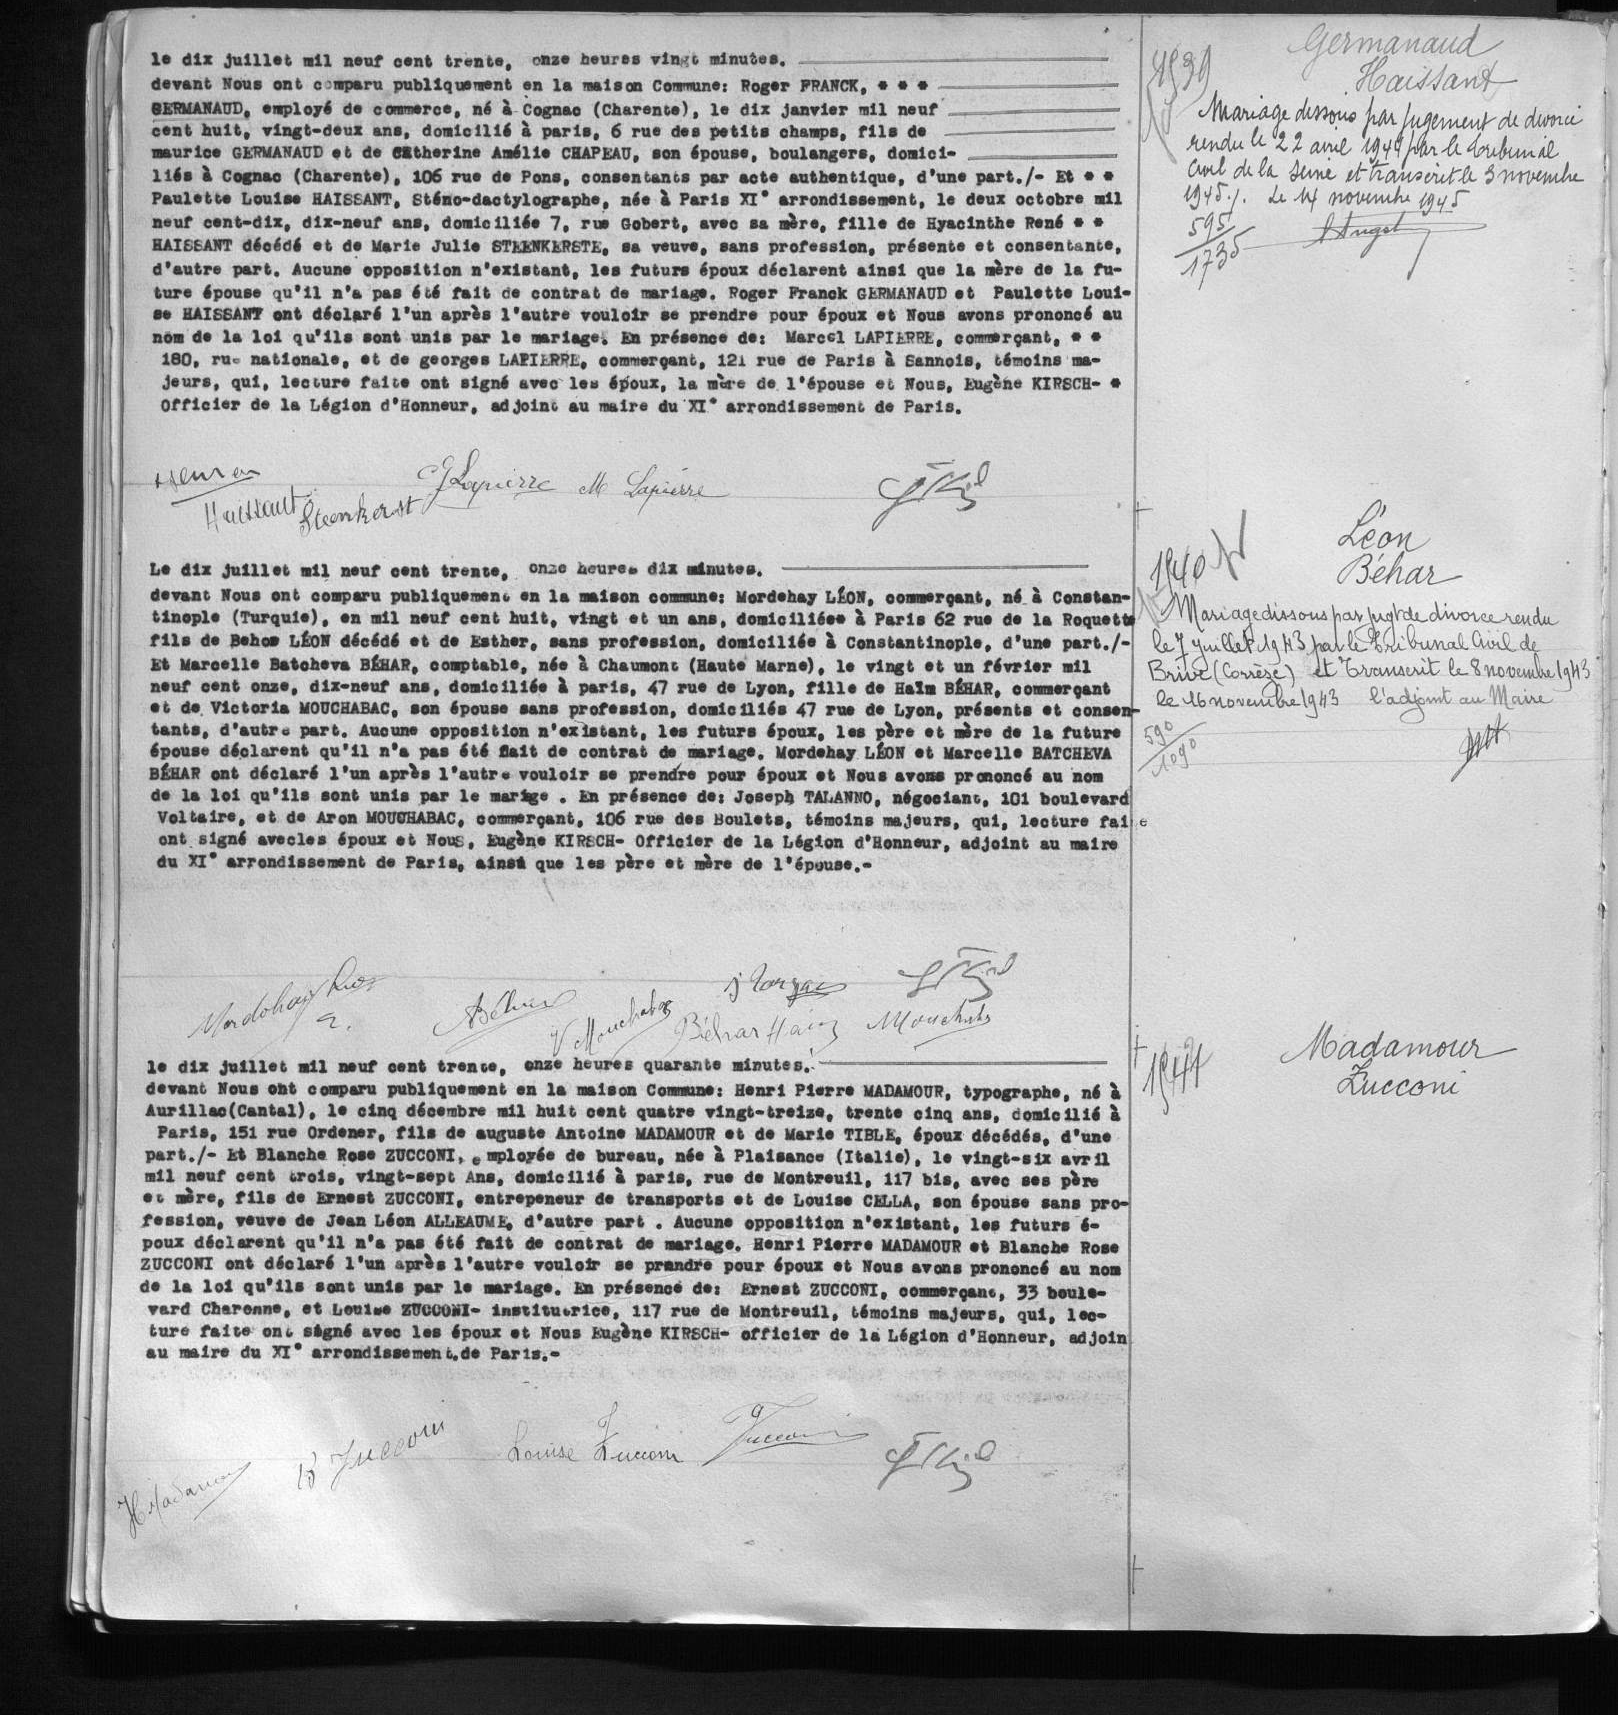le dix juillet mil neuf cent trente, onze heures vingt minutes .-
devant Nous ont comparu publiquement en la maison Commune: Roger FRANCK, ***-
GERMANAUD, employé de commerce, né à Cognac (Charente), le dix janvier mil neuf -
cent huit, vingt-deux ans, domicilié à paris, 6 rue des petits champs, fils de -
maurice GERMANAUD et de CEtherine Amélie CHAPEAU, son épouse, boulanger, domici -
liés à Cognac (Charente), 106 rue de Pons, consentants par acte authentique, d'une part ./-Et **
Paulette Louise HAISSANT, Sténo-dactylographe, née à Paris XI ° arrondissement, le deux octobre mil
neuf cent-dix, dix-neuf ans, domiciliée 7, rue Gobert, avec sa mère, fille de Hyacinthe René **
HAISSANT décédé et de Marie Julie STEEKERSTE, sa veuve, sans profession, présente et consentante,
d'autre part. Aucune opposition n'existant, les futurs époux déclarent ainsi que la mère de la fu-
ture épouse qu'il n'a pas été fait de contrat de mariage. Roger Franck GERMANAUD et Paulette Loui-
se HAISSANY ont déclaré l'un après l'autre vouloir se prendre pour époux et Nous avons prononcé au
nom de la loi qu'ils sont unis par le mariage. En présence de: Marcel LAPIERRE, commerçant, **
180, rue nationale, et de georges LAPIERRE, commerçant, 121 rue de Paris Sannois, témoins ma-
jeurs, qui, lecture faite, ont signé avec les époux, la mère de l'épouse et Nous, Eugène KIRSCH -*
Officier de la Légion d'Honneur, adjoint au maire du XI ° arrondissement de Paris.
Le dix juillet mil neuf cent trente, onze heures dix minutes, -
devant Nous ont comparu publiquemenc en la maison commune: Mordehay LEON, commerçant, né à Constan-
tinople (Turquie), en mil neuf cent huit, vingt et un ans, domiciliée à Paris 62 rue de la Roquette
fils de Behoe LEON décédé et de Esther, sans profession, domiciliée à Constantinople, d'une part ./-
Et Marcelle Batcheva BEHAR, comptable, née à Chaumont (Haute Marne), le vingt et un février mil
neuf cent onze, dix-neuf ans, domicilié à paris, 47 rue de Lyon, fille de Haïm BEHAR, commerçant
et de Victoria MOUCHABAC, son épouse sans profession, domiciliés 47 rue de Lyon, présents et consen
tants, d'autre part. Aucune opposition n'existant, les futurs époux, les père et mère de la future
épouse déclarent qu'il n'a pas été fait de contrat de mariage. Mordehay LEON et Marcelle BATCHEVA
BEHAR ont déclaré l'un après l'autre vouloir se prendre pour époux et Nous avons prononcé au nom
de la loi qu'ils sont unis par le mariage. En présence de: Joseph TALANNO, négociants, 101 boulevard
Voltaire, et de Aron MOUCHABAC, commerçant, 106 rue des Boulets, témoins majeurs, qui, lecture fai
ont signé avec les époux et Nous, Eugène KIRSCH-Officier de la Légion d'Honneur, adjoint au maire
du XI ° arrondissement de Paris, ains* que les père et mère de l'épouse .-
le dix juillet mil neuf cent trente, onze heures quarante minutes .-
devant Nous ont comparu publiquement en la maison Commune: Henri Pierre MADAMOUR, typographe, né à
Aurillac (Cantal), le cinq décembre mil huit cent quatre vingt-treize, trente cinq ans, domicilié à
Paris, 151 rue Ordener, fils de auguste Anotine MADAMOUR et de Marie TIBLE, époux décédés, d'une
part ./-Et Blanche ROSE ZUCCONI, employée de bureau, née à Plaisance (Italie), le vingt-six avril
mil neuf cent trois, vingt-sept Ans, domicilié à paris, rue de Montreuil, 117 bis, avec ses père
et mère, fils de Ernest ZUCCONI, entrepreneur de transports et Louise CELLA, son épouse sans pro-
fession, veuve de Jean Léon ALLEAUME, d'autre part. Aucune opposition n'existant, les futurs é-
poux déclarent qu'il n'a pas été fait de contrat de mariage. Henri Pierre MADAMOUR et Blanche Rose
ZUCCONI ont déclaré l'un après l'autre vouloir se prendre pour époux et Nous avons prononcé au nom
de la loi qu'ils sont unis par le mariage. En présence de: Ernest ZUCCONI, commerçant, 33 boule-
vard Charonne, et Louise ZUCCONI-institutrice, 117 rue de Montreuil, témoins majeurs, qui, lec-
ture faite ont signé avec les époux et Nous Eugène KIRSCH-officier de la Légion d'Honneur, adjoint
au maire du XI° arrondissement. de Paris.-
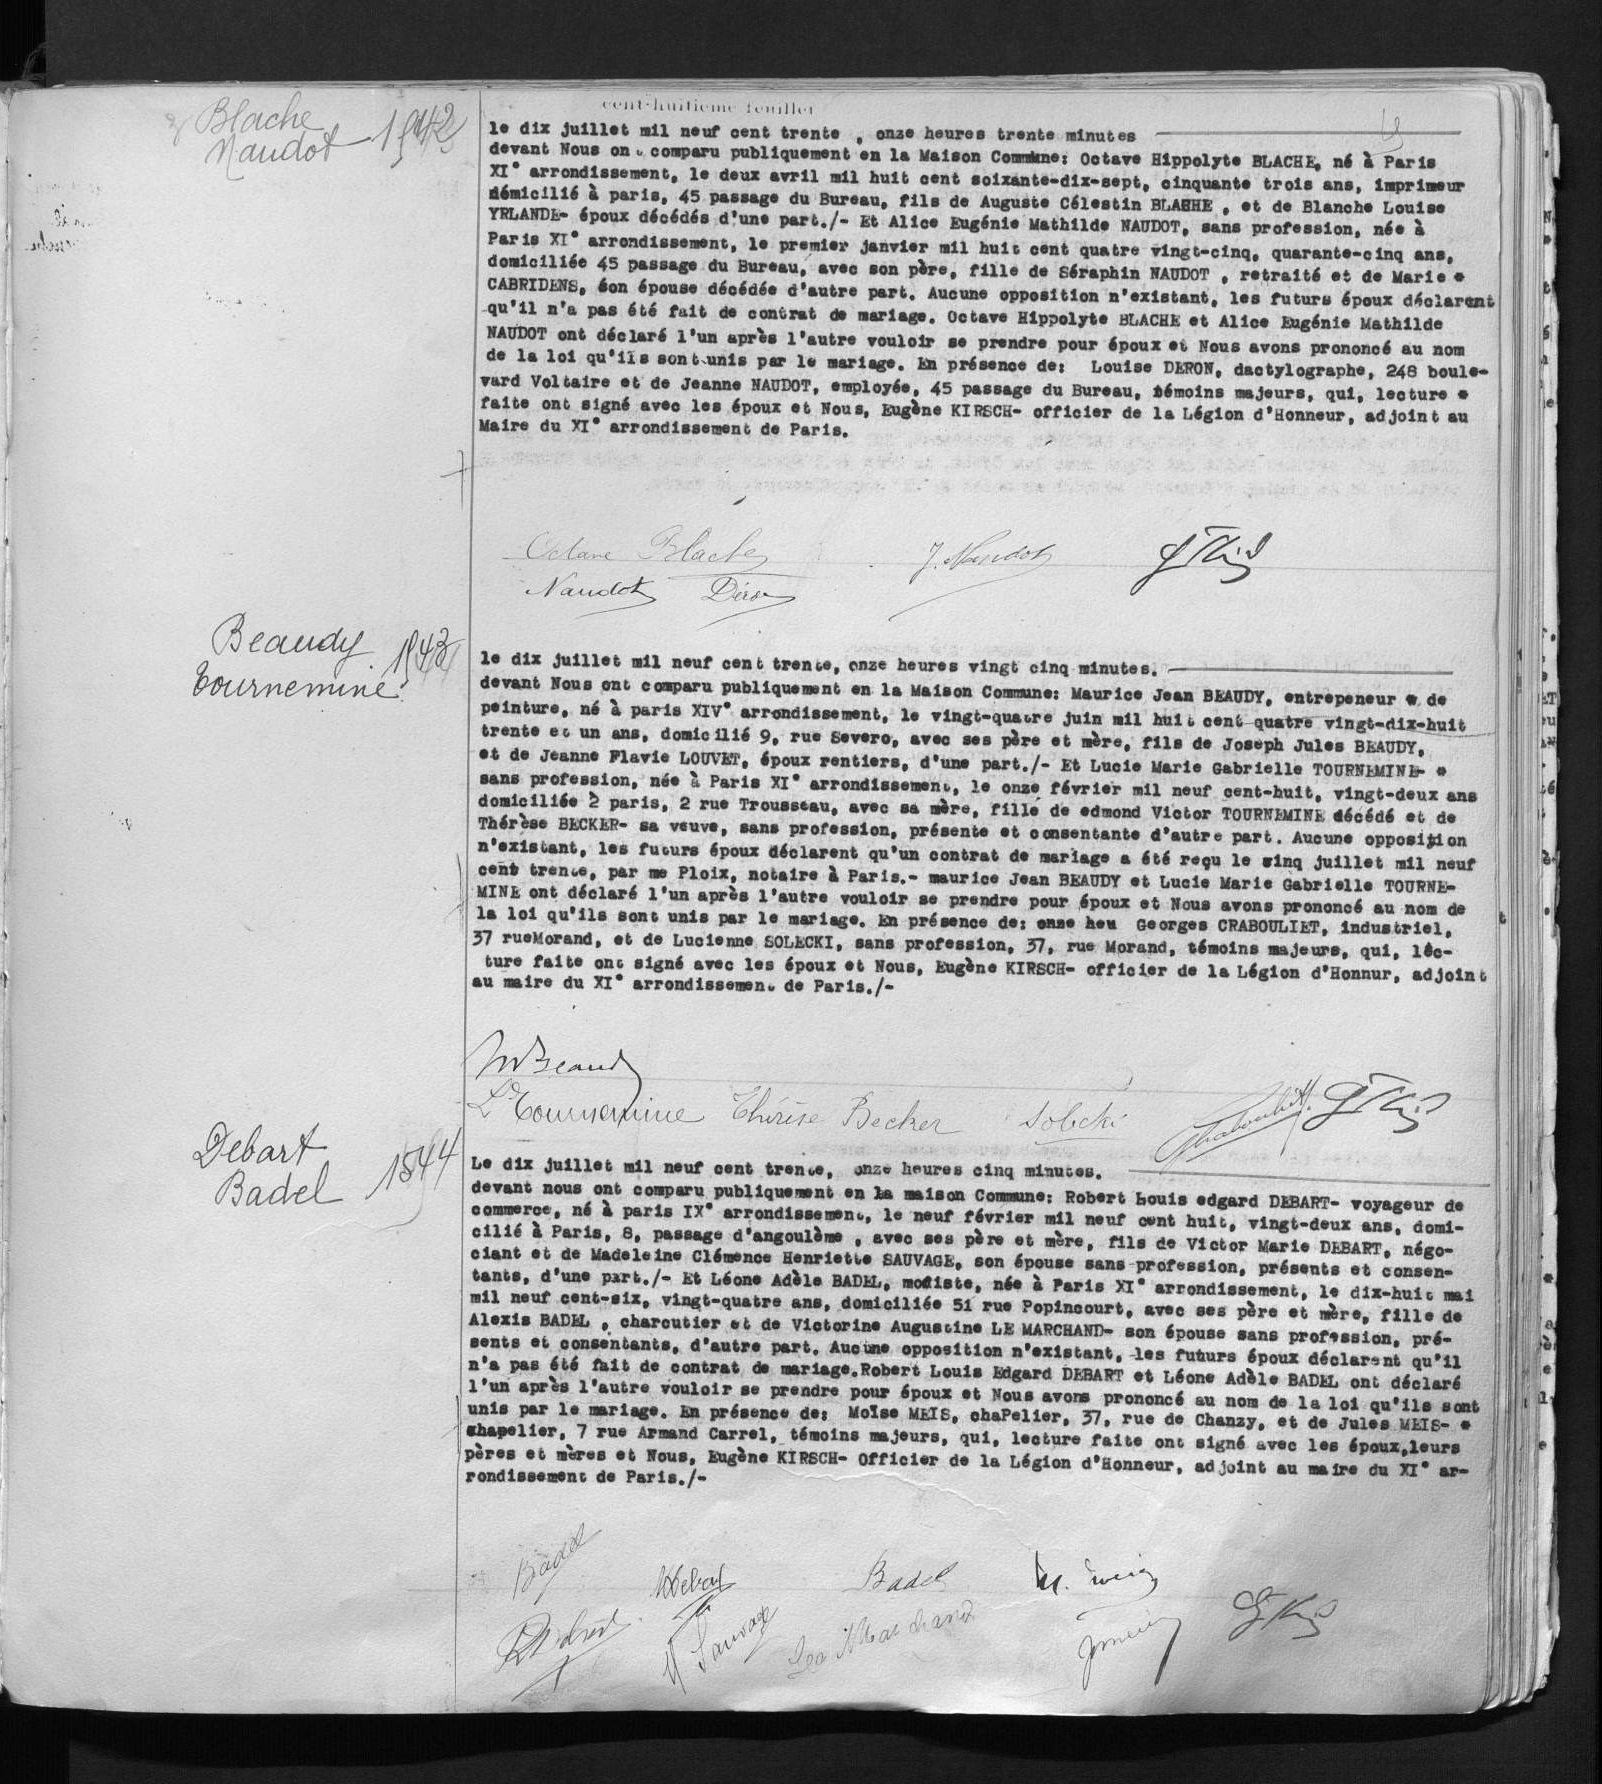le dix juillet mil neuf cent trente, onze heures trente minutes-
devant Nous on comparu publiquement en la Maison Commune: Octave Hippolyte BLACHE, né à Paris
XI ° arrondissement, le deux avril mil huit cent soixante-dix-sept, cinquante trois ans, imprimeur
domicilié à paris, 45 passage du Bureau, fils de Auguste Célestin BLABHE, et de Blanche Louise
YRLANDE-époux décédés d'une part ./-Et Alice Eugénie Mathilde NAUDOT, sans profession, née à
Paris XI ° arrondissement, le premier janvier mil huit cent quatre vingt-cinq, quarante-cinq ans,
domiciliée 45 passage du Bureau, avec son père, fille de Séraphin NAUDOT, retraité et de Marie *
CABRIDENS, son épouse décédée d'autre part. Aucune opposition n'existant, les futurs époux déclarent
qu'il n'a pas été fait de contrat de mariage. Octave Hippolyte BLACHE et Alice Eugénie Mathilde
NAUDOT ont déclaré l'un après l'autre vouloir se prendre pour époux et Nous avons prononcé au nom
de la loi qu'ils sont unis par le mariage. En présence de: Louise DERON, dactylographe, 248 boule-
vard Voltaire et de Jeanne NAUDOT, employée, 45 passage du Bureau, témoins majeurs, qui, lecture *
faite ont signé avec les époux et Nous, Eugène KIRSCH-officier de la Légion d'Honneur, adjoint au
Maire du XI ° arrondissement de Paris.
le dix juillet mil neuf cent trente, onze heures vingt cinq minutes.-
devant Nous ont comparu publiquement en la Maison Commune: Maurice Jean BEAUDY, entrepreneur * de
peinture, né à paris XIV ° arrondissement, le vingt-quatre juin mil hui cent quatre vingt-dix-huit
trente et un ans, domicilié 9, rue Severo, avec ses père et mère, fils de Joseph Jules BEAUDY,
et de Jeanne Flavie LOUVET, époux rentiers, d'une part ./-Et Lucie Marie Gabrielle TOURNEMINE -
sans profession, née à Paris XI ° arrondissement, le onze février mil neuf cent-huit, vingt-deuxans
domiciliée 2 paris, 2 rue Trousseau, avec sa mère, fille de edmond Victor TOURNEMINE décédé et de
Thérèse BECKER-sa veuve, sans profession, présente et consentante d'autre part. Aucune opposition
n'existant, les futurs époux déclarent qu'un contrat de mariage a été reçu le vinq juillet mil neuf
cent trente, par me Ploix, notaire à Paris .-maurice Jean BEAUDY et Lucie Marie Gabrielle TOURNE-
MINE ont déclaré l'un après l'autre vouloir se prendre pour époux et Nous avons prononcé au nom de
la loi qu'ils sont unis par le mariage. En présence de: enne heu Georges CRABOULIET, industriel,
37 rue Morand, et de Lucienne SOLECKI, sans profession, 37, rue Morand, témoins majeurs, qui, lec-
ture faite ont signé avec les époux et Nous, Eugène KIRSCH-officier de la Légion d'Honneur, adjoint
au maire du XI ° arrondissemen de Paris ./-
Le dix juillet mil neuf cent trente, onze heures cinq minutes, -
devant nous ont comparu publiquement en la maison Commune: Robert Louis edgard DEBART-voyageur de
commerce, né à paris IX ° arrondissemen, le neuf février mil neuf cent huit, vingt-deux ans, domi-
cilié à Paris, 8, passage d'angoulème, avec ses père et mère, fils de Victor Marie DEBART, négo-
ciant et de Madeleine Clémence Henriette SAUVAGE, son épouse sans profession, présents et consen-
tants, d'une part ./-Et Léone Adèle BADEL, modiste, née à Paris XI ° arrondissement, le dix-huit mai
mil neuf cent-six, vingt-quatre ans, domiciliée 51 rue Popincourt, avec ses père et mère, fille de
Alexis BADEL, charcutier et de Victorine Augustine LE MARCHAND-son épouse sans profession, pré-
sents et consentants, d'autre part. Aucune opposition n'existant, les futurs époux déclarent qu'il
n'a pas été fait de contrat de mariage. Robert Louis Edgard DEBART et Léone Adèle BADEL ont déclaré
l'un après l'autre vouloir se prendre pour époux et Nous avons prononcé au nom de la loi qu'ils sont
unis par le mariage. En présence de: Moïse MEIS, chaPelier, 37, rue de Chanzy, et de Jules MEIS -*
chapelier, 7 rue Armand CARREL, témoins majeurs, qui, lecture faite ont signé avec les époux, leurs
pères et mères et Nous, Eugène KIRSCH-Officier de la Légion d'Honneur, adjoint au maire du XI ° ar-
rondissement de Paris ./-